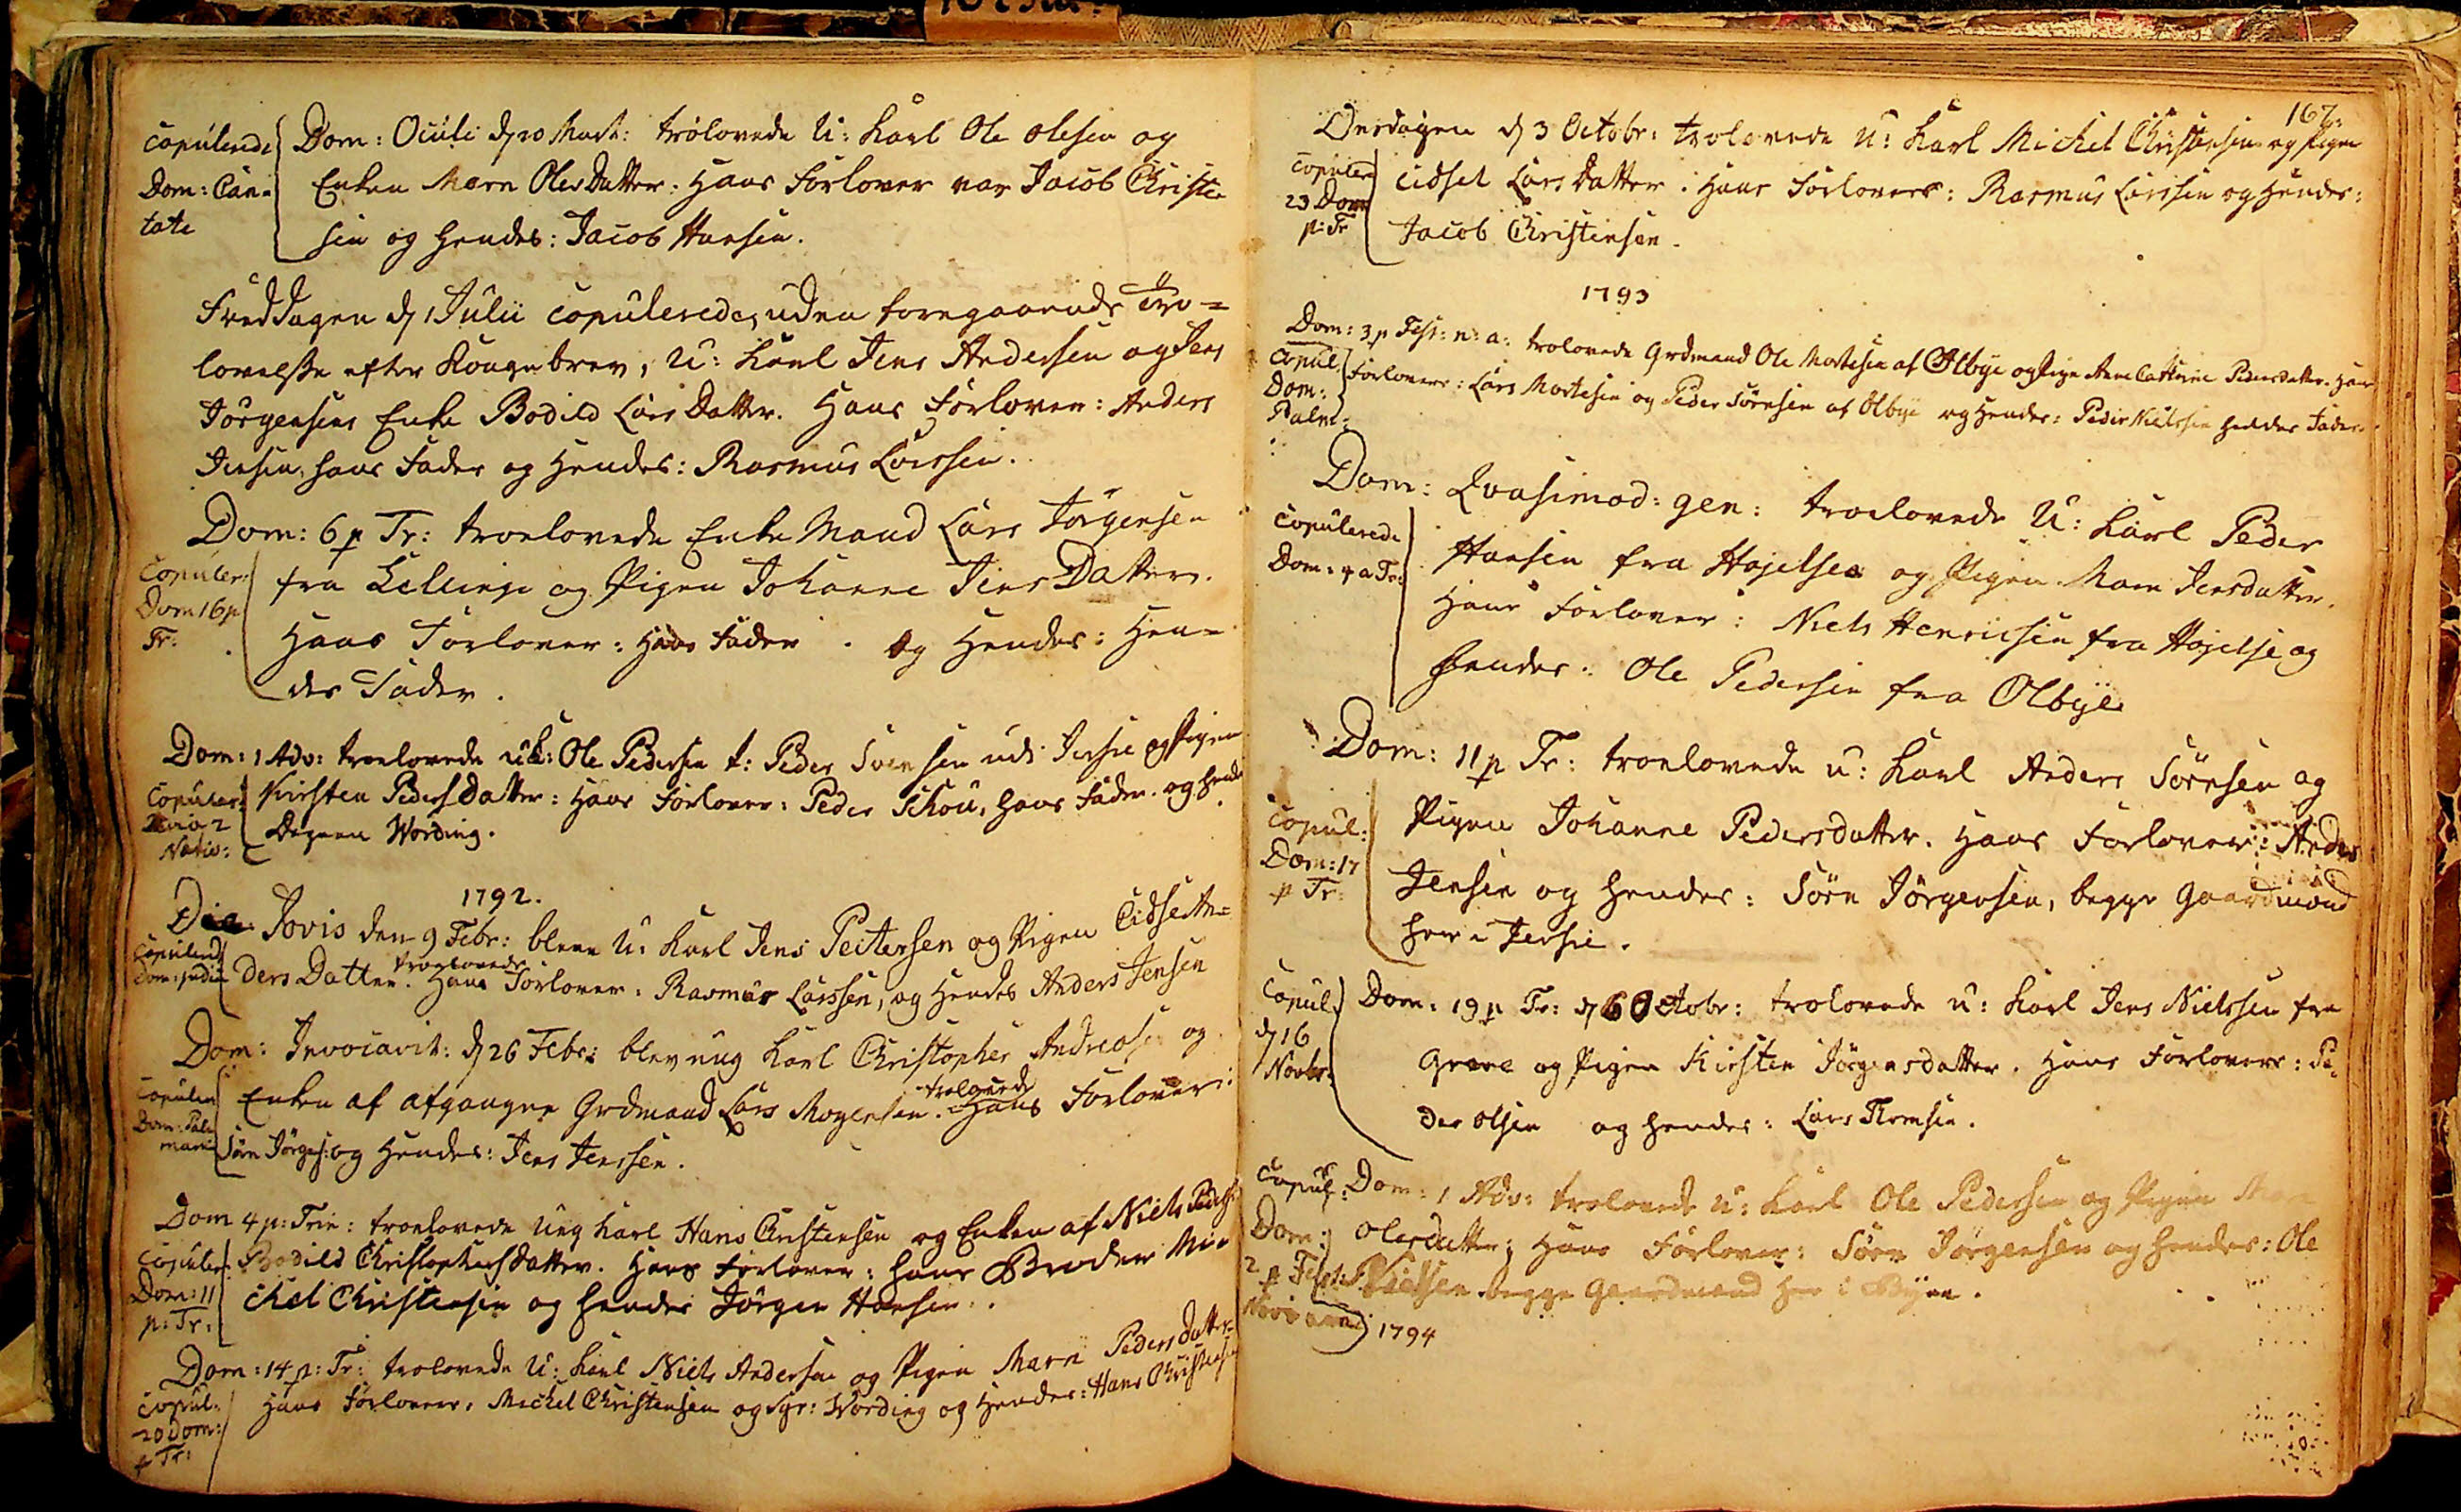Dom: Oculi d 20 Mart: trolovede U: karl Ole Olesen og
Enken Marn Oles Datter: Hans Forlover var Jacob Christen
sen og hendes: Jacob Hansen.
Copulerede
Dom: Can¬
tate
Freddagen d 1 Julii copuleredes uden foregaaende Tro¬
lovelse efter Kongebrev, U:karl Jens Andersen og Jens
Jørgensens Enke Bodild Lars Datter. Hans Forlover: Anders
Jensen, hans Fader og Hendes: Rasmus Larssen.
Dom: 6 p Tr: troelovede Enke Mand Lars Jørgensen
fra Lellinge og Pigen Johanne Jens Datter.
Hans Forlover: Hans Fader
og Hendes: Hen¬
des Fader.
Copuler:
Dom 16 p
Tr: 
Dom: 1 Adv: troelovede uk: Ole Pedersen t: Peder Svensen udi Jersie og Pigen
Kirsten Pedersdatter. Hans Forlover: Peder Schou, hans Fader. og hendes
Degnen Wording.
Copuler:
Feria 2
Nativ:
1792.
Die: Jovis den 9 Febr: bleve U: karl Jens Peitersen og Pigen Cidse An¬
troelovede
ders Datter. Hans Forlover: Rasmus Larsen, og Hendes Anders Jensen
Copulered
Dom: Judic
Dom: Invocavit: d 26 Febr: blev ung karl Christopher Andreæsen og
trolovede
Enken af afgangne Grdmand Lars Mogensen. Hans Forlover:
Sørn Jørgns: og Hendes: Jens Jenssen.
Copuler
Dom: Palm
marum
Dom: 4 p Trin: troelovede Ung karl Hans Christensen og Enken af Niels Peders:
Bodild Christophersdatter. Hans Forlover: hans Broder Mi¬
chel Christensen og hendes Jørgen Hansen.
Copuler.
Dom: 11
p: Tr:
Dom: 14 p Tr: trolovede U: karl Niels Andersen og Pigen Marn Pedersdatter.
Hans forlover: Michel Christensen og Sgr: Wording og Hendes: Hans Christensen
Copul:
20 Dom:
p Tr:
167.
Onsdagen d 3 Octobr: trolovede U: karl Michel Christensen og Pigen
Cidsel Lars Datter: Hans Forlovere: Rasmus Larsen og Hendes:
Jacob Christensen.
Copulered
23 Dom
p: Tr
1793
Dom: 3 p Fest: n:a: trolovede Grdmand Ole Mortesen af Ølbye og Pige Anne Catrine Pedersdatter. Hans
Forlovere: Lars Mortesen og Peder Sørnsen af Ølbye og Hendes: Peder Nielssen hendes Fader.
Copul:
Dom:
Palm:
Dom: Qvasimod: gen: troelovede U: karl Peder
Hansen fra Højelse og Pigen Marn Jensdatter.
Hans Forlover: Niels Henricsen fra Højelse og
hendes: Ole Pedersen fra Ølbye
Copulerede
Dom:4 a Tr:
Dom: 11 p Tr: troelovede u: karl Anders Sørnsen og
Pigen Johanne Pedersdatter. Hans Forlover: Anders
Jensen og hendes: Sørn Jørgensen, begge Gaardmænd
her i Jersie.
Copul:
Dom: 17
p Tr:
Dom: 19 p Tr: d 6 Octobr: trolovede u: karl Jens Nielssen fra
Greve og Pigen Kirsten Jørgensdatter. Hans Forlovere: Pe
der Olsen og hendes: Lars Thomsen.
Copul:
d 16
Novbr:
Dom: 1 Adv: trolovede u: karl Ole Pedersen og Pigen Marn
Olesdatter; Hans Forlover: Sørn Jørgensen og hendes: Ole
Nielsen. begge Gaardmænd her i Byen.
Copul:
Dom:
2 p Fest:
Novi Anni 1794 
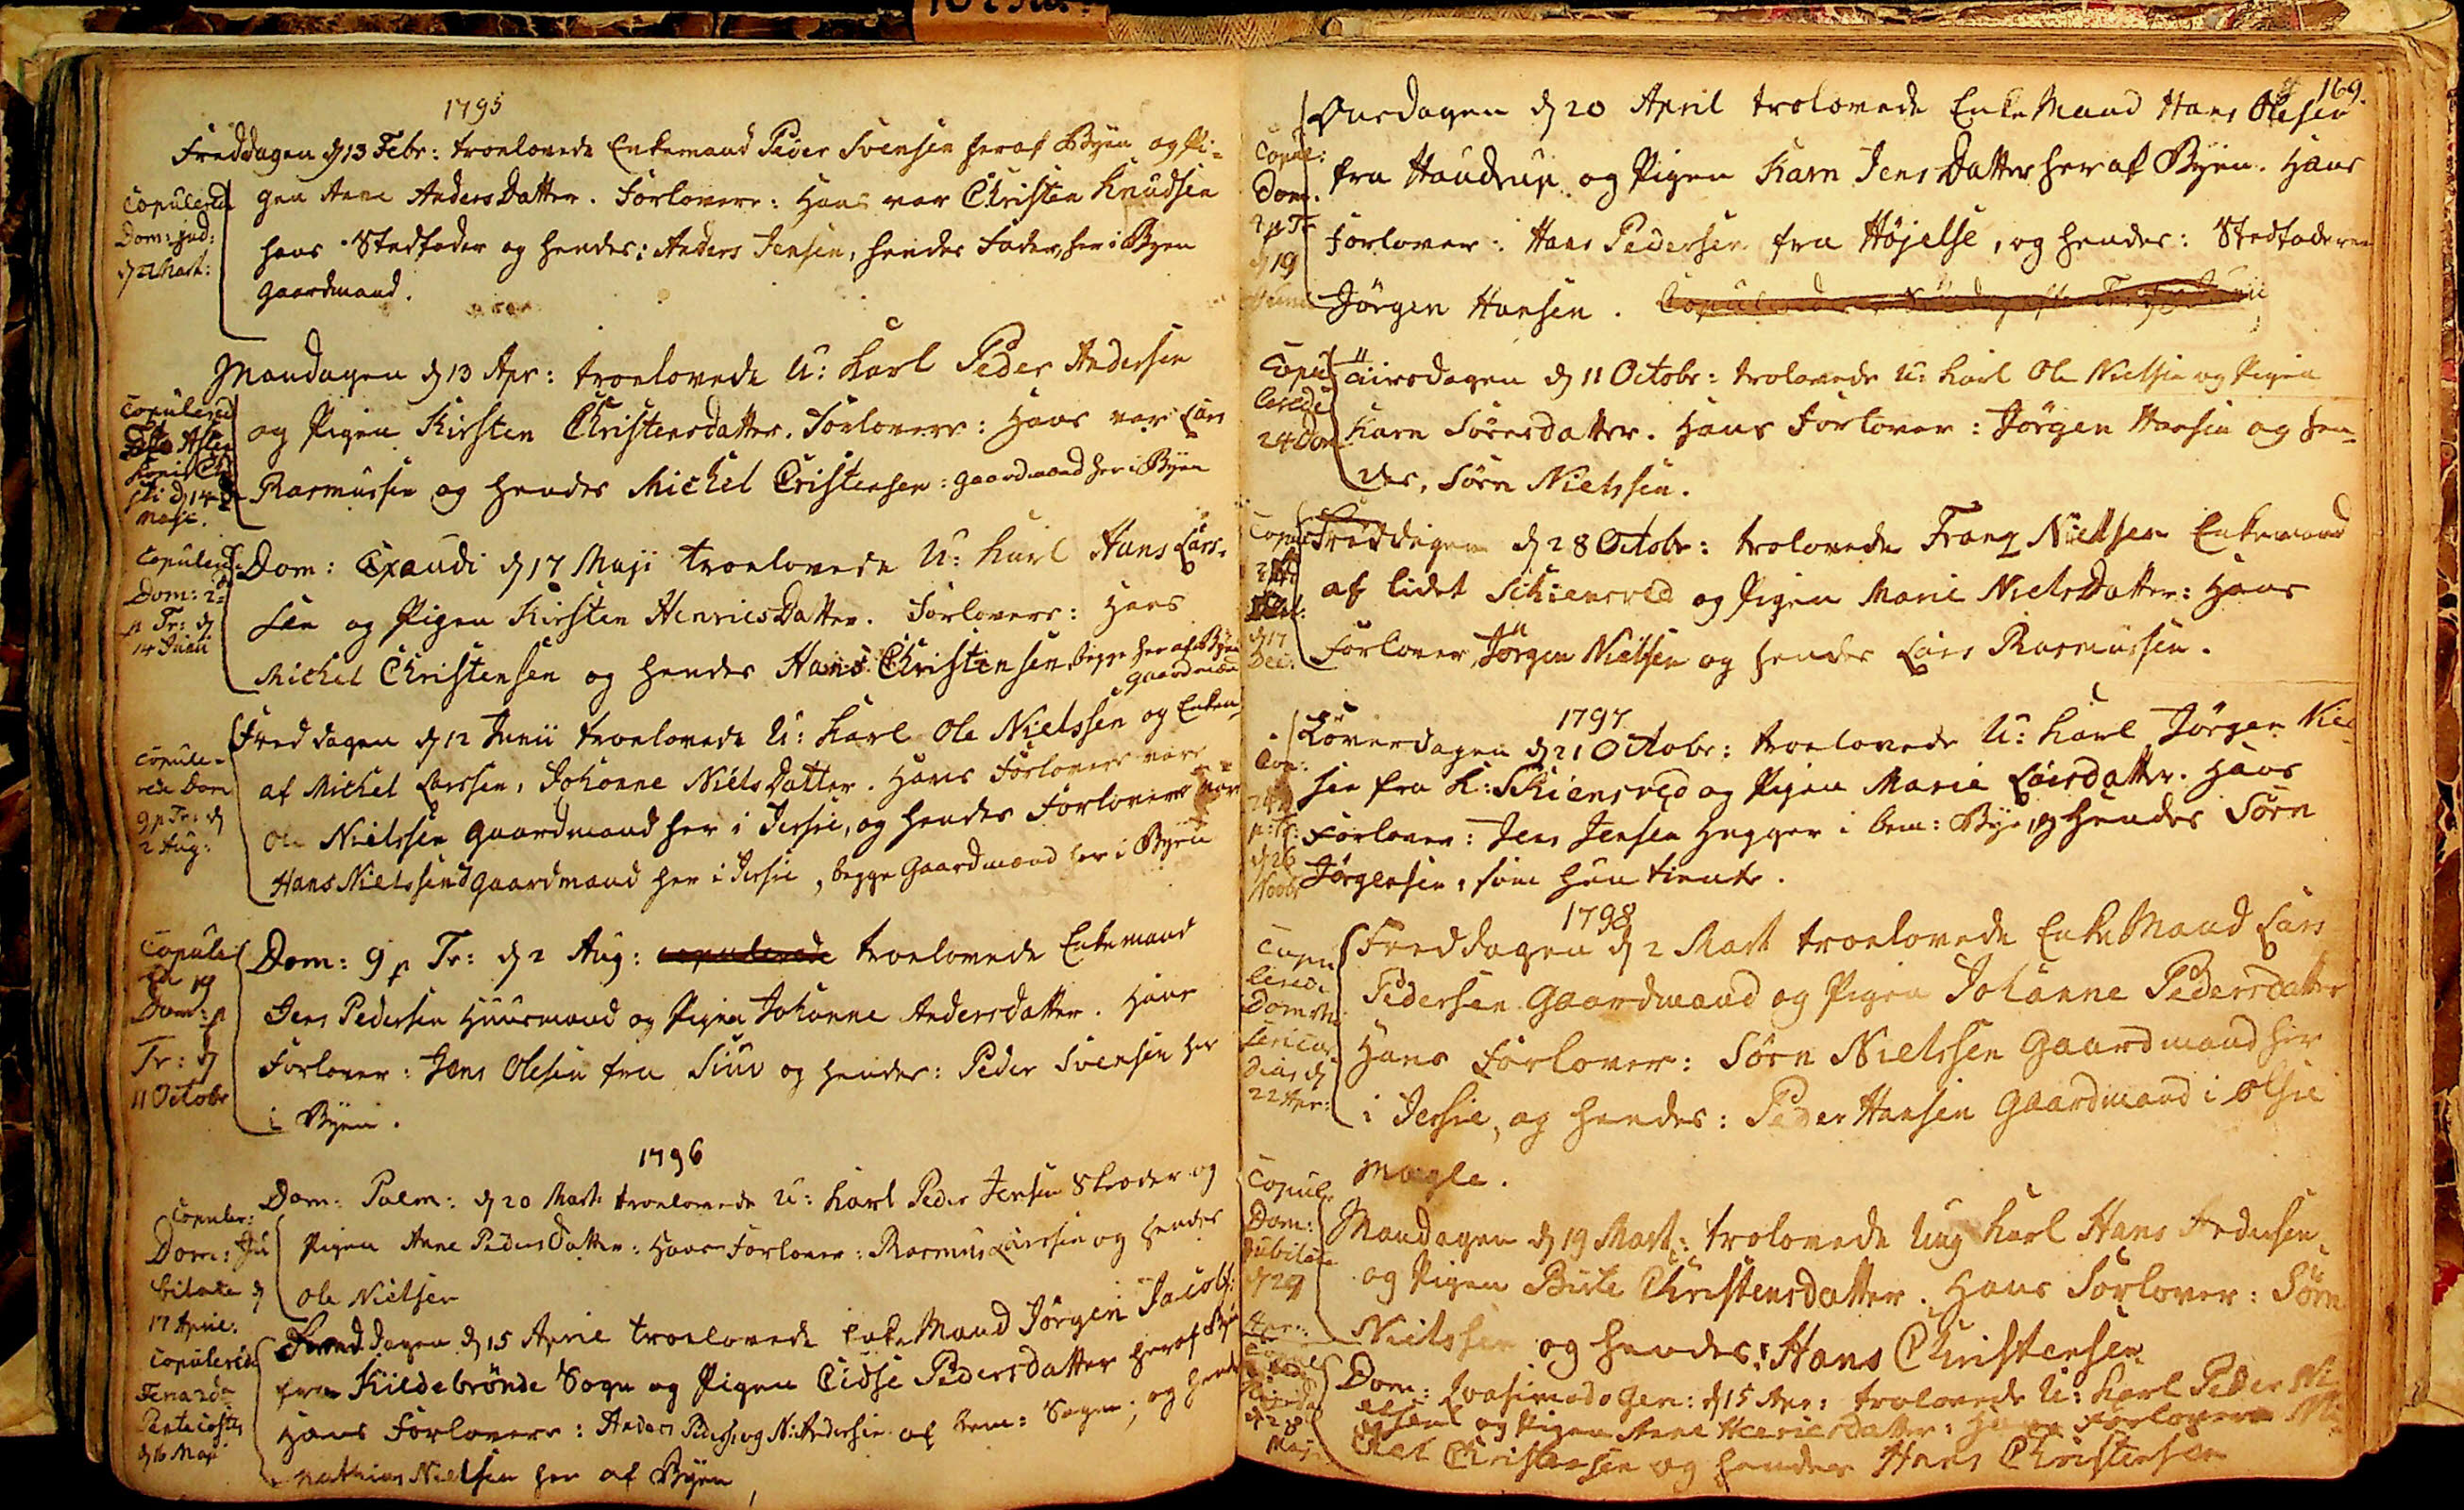1795
Freddagen d 13 Febr: troelovede Enkemand Peder Svensen heraf Byen og Pi¬
gen Anne Anders Datter. Forlovere: Hans var Christen Knudsen
hans Stedfader og hendes: Anders Jensen, hendes Fader, her i Byen
Gaardmand.
Copulerede
Dom: jud:
d 22 Mart:
Mandagen d 13 Apr: troelovede U: karl Peder Andersen
og Pigen Kirsten Christensdatter. Forlovere: Hans var Lars
Rasmussen og hendes Michel Christensen: Gaardmænd her i Byen
Copulerede
Festo Ascen
sionis Chri
sti d 14de
Maji.
Dom: Exaudi d 17 Maji troelovede U: karl Hans Lars¬
sen og Pigen Kirsten HenricsDatter. Forlovere: Hans
Michel Christensen og hendes Hans Christensen. Begge her af Byen
Gaardmænd
Copulerede
Dom: 2da
p Tr: d
14 Junii
Freddagen d 12 Junii troelovede U: karl Ole Nielssen og Enken
af Michel Larssen, Johanne Niels Datter. Hans Forlovere vare
Ole Nielssen Gaardmand her i Jersie, og hendes Forlovere vare
Hans Nielssen og Gaardmand her i Jersie, begge Gaardmænd her i Byen
Copule¬
rede Dom
9 p Tr: d
2 Aug:
Dom: 9 p Tr: d 2 Aug: copulerede troelovede Enkemand
Jens Pedersen Huusmand og Pigen Johanne Andersdatter. Hans
Forlover: Jens Olesen fra Siuv og hendes: Peder Svensen her
i Byen
Copule
rede 19
Dom: p
Tr: d
11 Octobr
1796
Dom: Palm: d 20 Mart: troelovede u: karl Peder Jensen Skræder og
Pigen Anne Pedersdatter: Hans Forlover: Rasmus Larssen og hendes
Ole Nielsen
Copuler:
Dom: Ju
bilate d
17 April.
Freddagen d 15 April Troelovede Enke Mand Jørgen Jacobs:
fra Kildebrønde Sogn og Pigen Cidse Pedersdatter heraf Byen
Hans Forlovere: Anders Peders: og N: Andersen af bem: Sogn; og hendes
Mathias Nielsen her af Byen,
Copulerede
Feria 2da
Pentacoste
d 16 Maji
169.
Onsdagen d 20 April trolovede Enkemand Hans Olesen
fra Haudrup og Pigen Karn Jens Datter her af Byen. Hans
Forlover: Hans Pedersen fra Højelse, og hendes: Stedfaderen
Jørgen Hansen.
Copul:
Dom:
4 p Tr
d 19
Junii
Tiirsdagen d 11 Octobr: trolovede U: karl Ole Nielsen og Pigen
Karn Sørnsdatter. Hans Forlover: Jørgen Hansen og hen¬
des, Sørn Nielssen.
Copu
lerede
24 Dom
Freddagen d 28 Octobr: trolovede Franz Nielsen Enkemand
af lidet Schiensved og Pigen Marie NielsDatter: Hans
Forlover, Jørgen Nielsen og hendes Lars Rasmussen.
Copul
3 Ad
d 11
Dec:
1797
Løverdagen d 21 Octobr: trolovede U:karl Jørgen Niel¬
sen fra K:Schiensved og Pigen Marie Larsdatter. Hans
Forlovere: Jens Jensen Hugger i Bem: Bye, og hendes Sørn
Jørgensen, som hun tiente.
Cop:
24 D:
p: Tr:
d 26
Novbr
1798
Freddagen d 2 Mart troelovede EnkeMand Lars
Pedersen Gaardmand og Pigen Johanne Pedersdatter
Hans Forlover: Sørn Nielssen Gaardmand her
i Jersie, og hendes: Peder Hansen Gaardmand i Ølsie
magle.
Copu
lerede
Dom Mi
sericor
dias d
22 Apr:
Mandagen d 19 Mart: trolovede Ung karl Hans Andersen
og Pigen Birte Christensdatter. Hans Forlover: Sørn
Nielssen og hendes: Hans Christensen
Copul:
Dom:
Jubilate
d 29
Apr:
Dom: Qvasimodo Gen: d 15 Apr: trolovede U:karl Peder Ni
elsen og Pigen Anne Henricsdatter: Hans Forlovere Mi¬
chel Christensen og hendes Hans Christensen
Copul
rede
Pinsdag
d 28
Maji